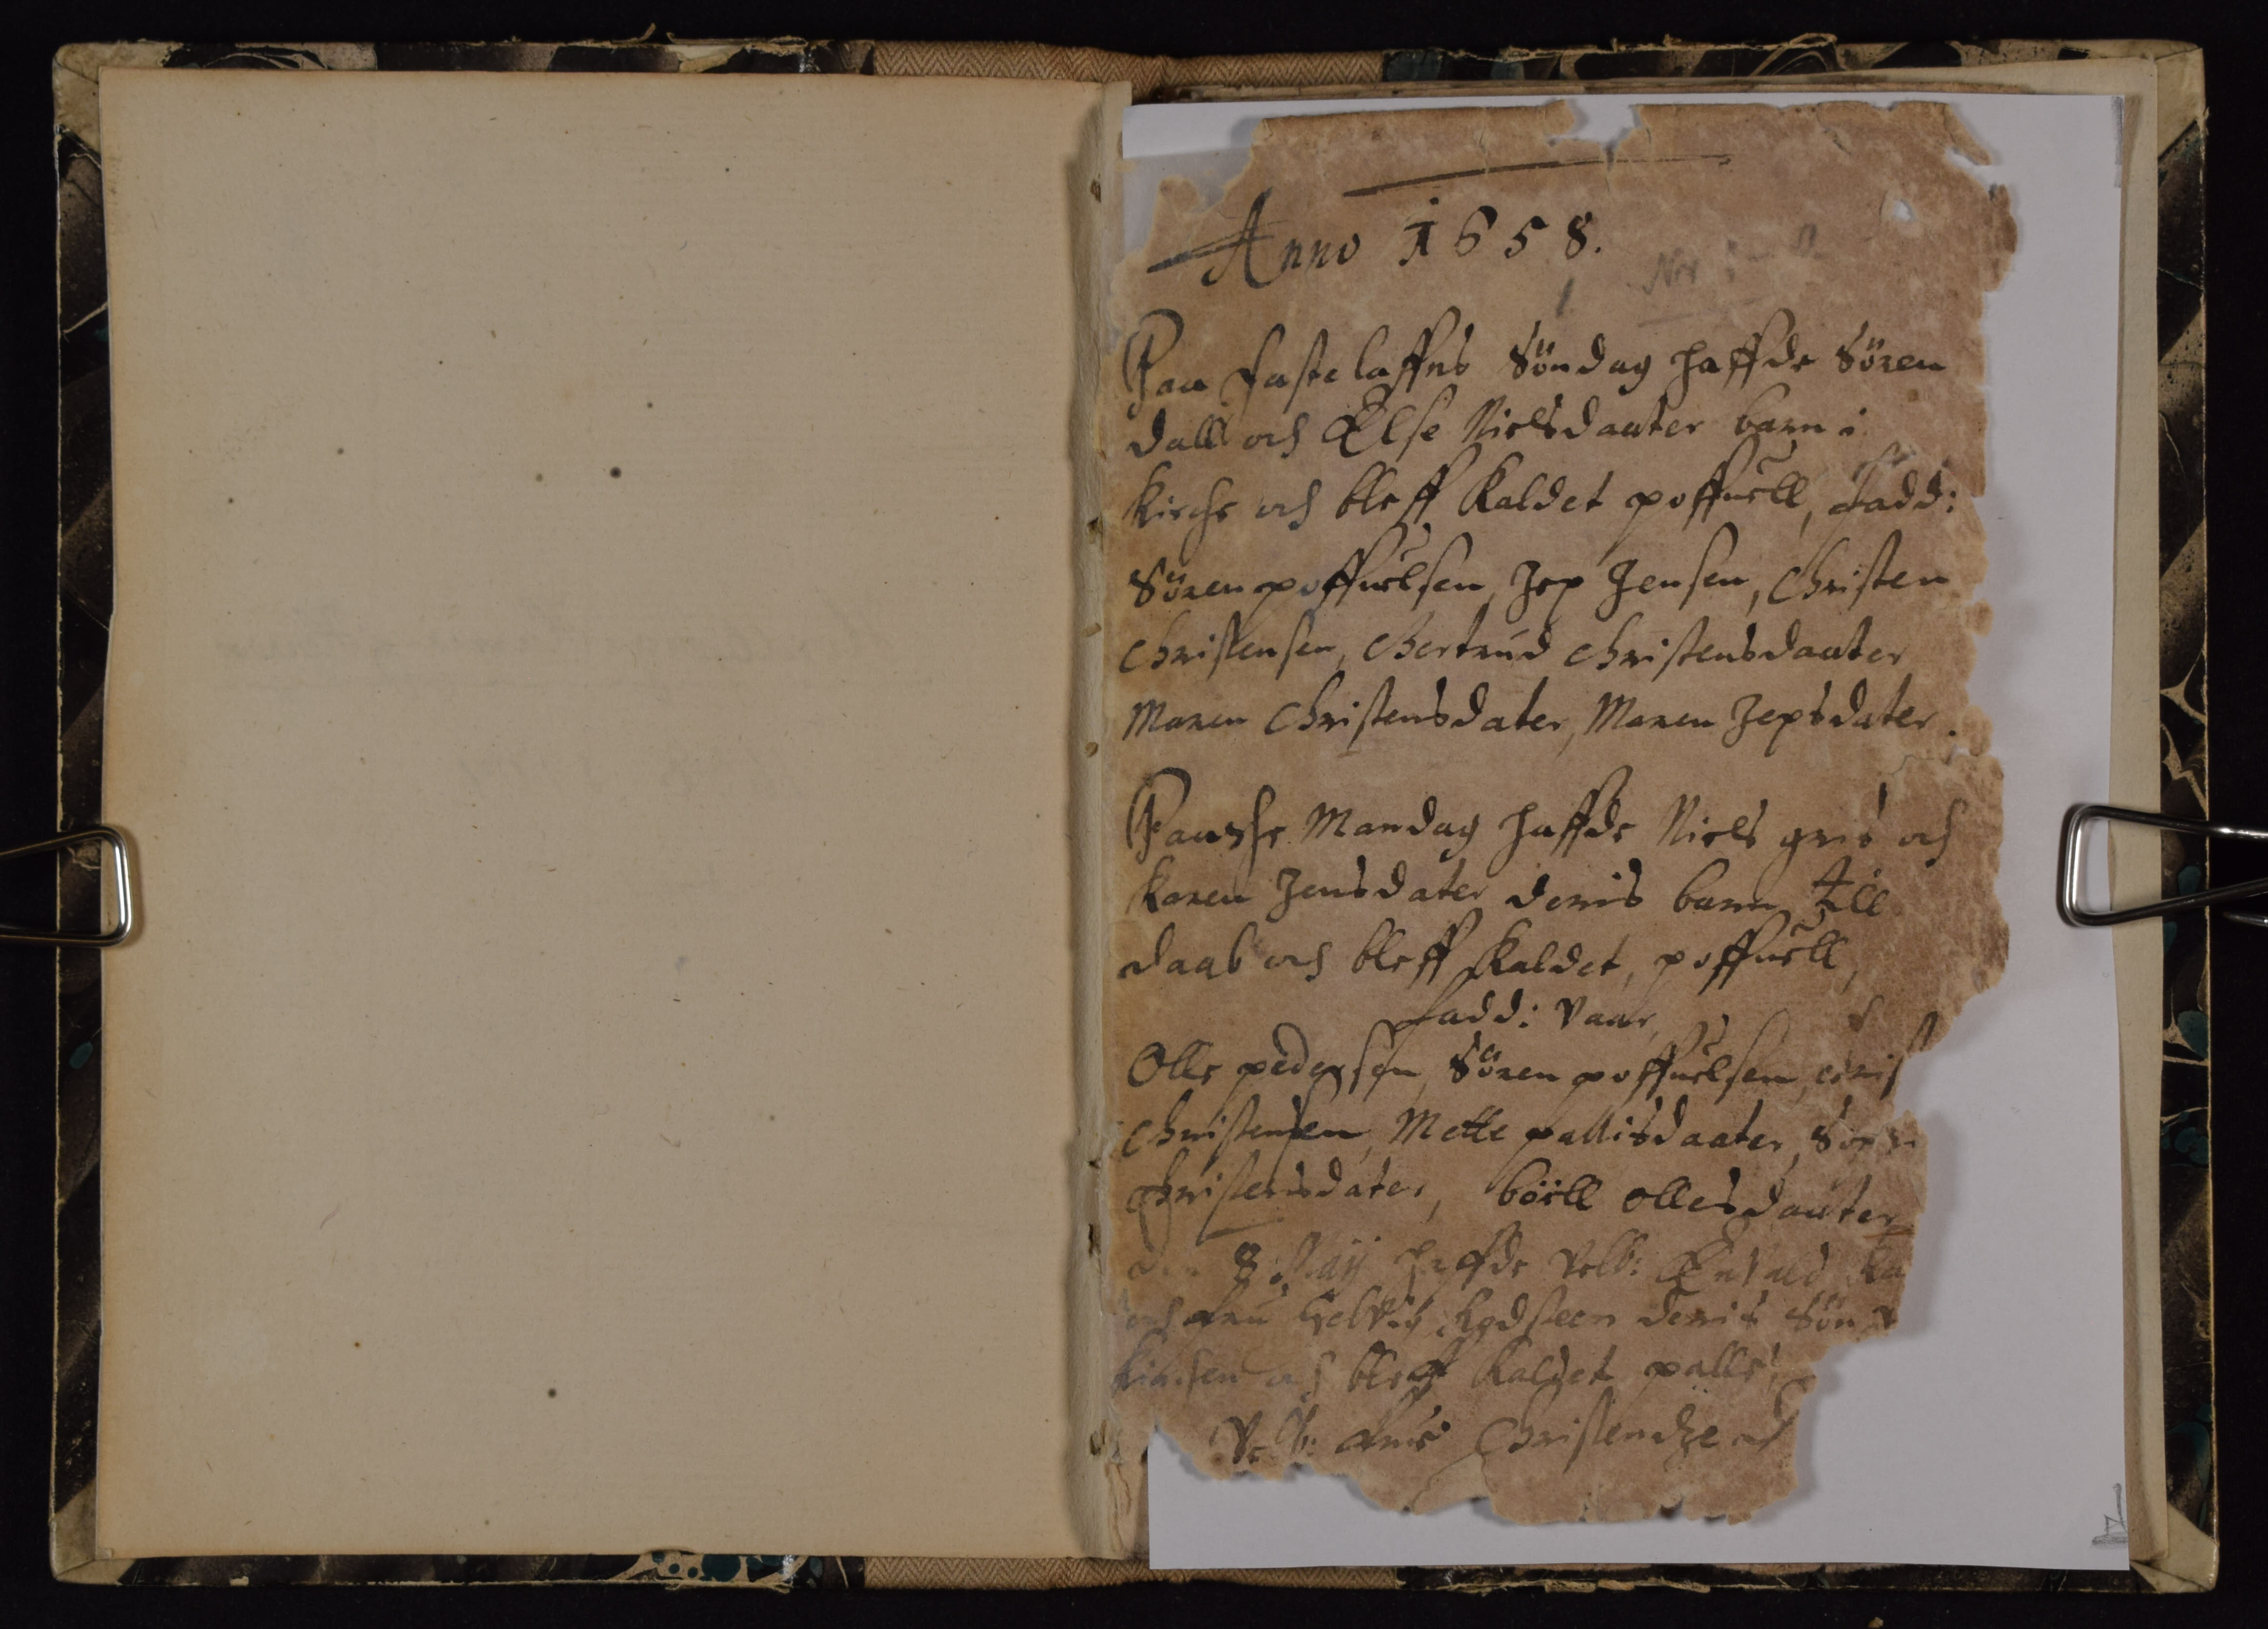Anno 1658.
Paa Fastelaffns Søndag haffde Søren
Dall och Else Nielsdauter barn i
Kirche och bleff kaldet poffuell Fadd:
Søren Poffuelsen, Jep Jensen, Christen
Christensen, Gertrud Christensdaater
Maren Christensdater, Maren Jepsdater
Pasche Mandag haffde Niels gr## och
Karen Jensdater deris barn till
daab och bleff kaldet, poffuell
Fadd: vaar
Olle pedersen Søren poffuelsen ##
Christensen Mette pallisdaater Sop##
Christensdater, boell Ollesdauter
Den 8 Maij hafde Velb: Envold Ka
och fru Helvig Radsteen deris Søn
Kirchen och bleff kaldet palle
Velb: fru Christendze
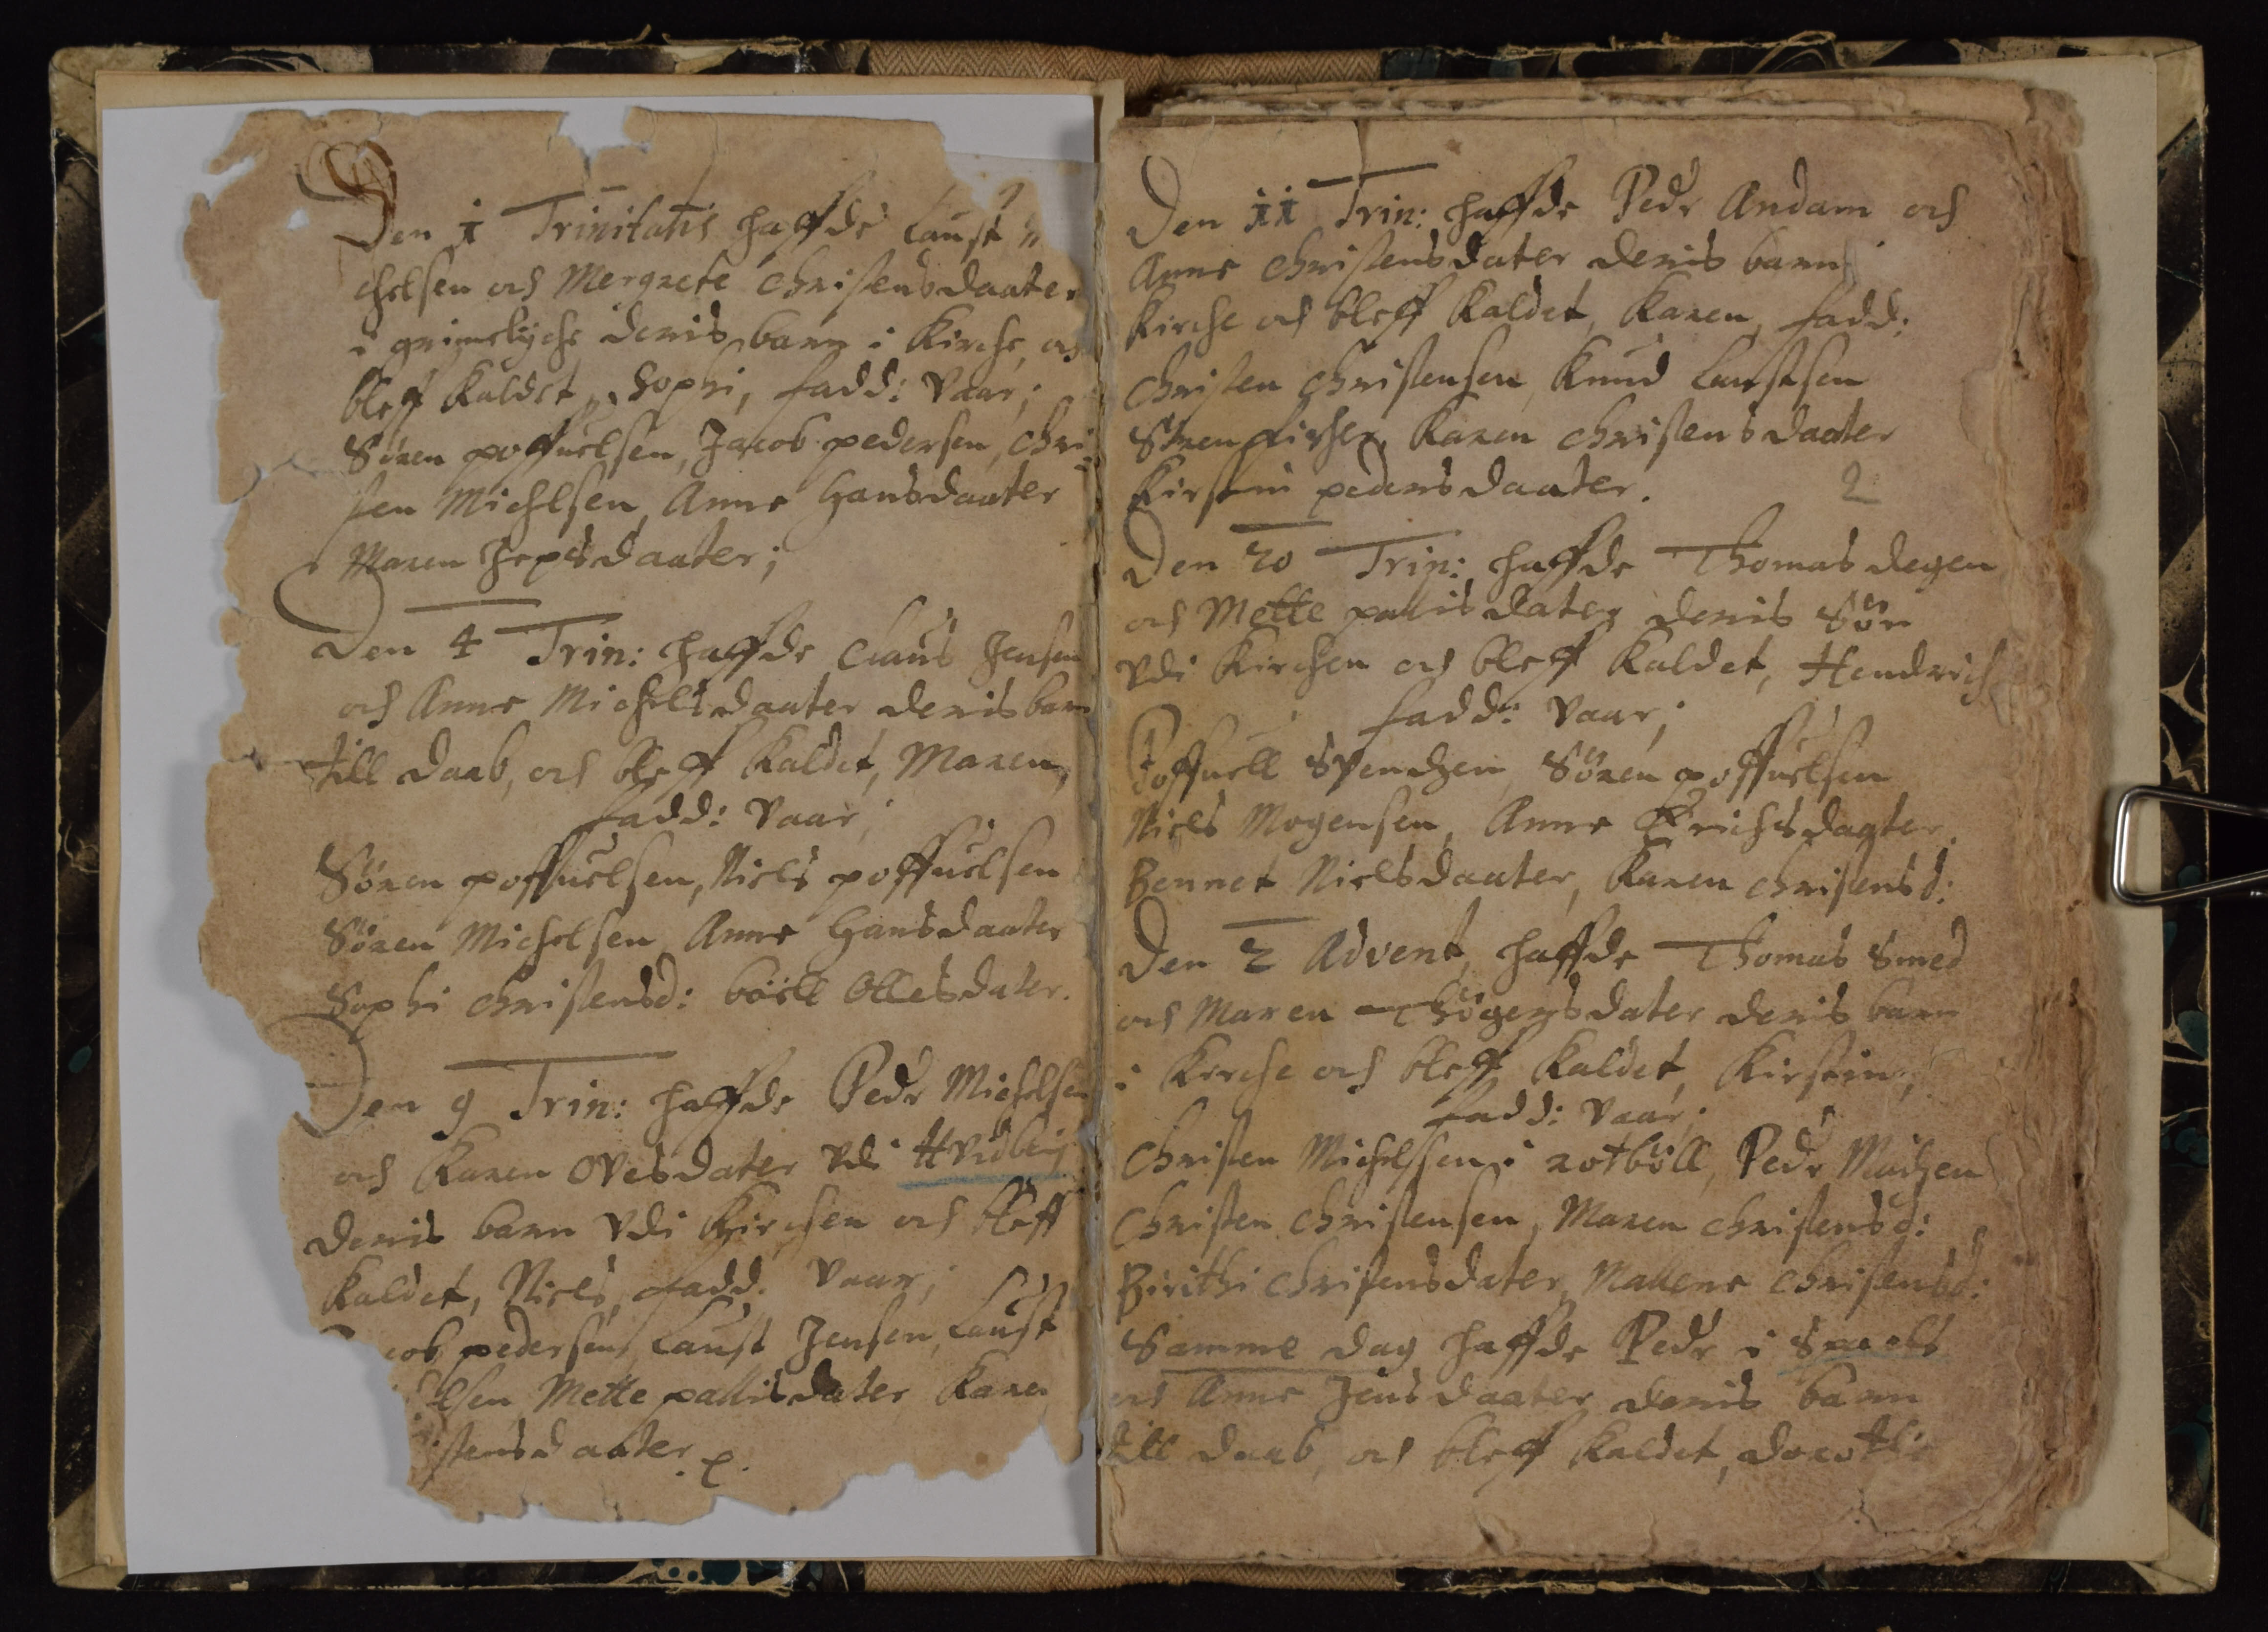Dom 1 Trinitatis haffde Laust
chelsen och Mergrete Christensdaater
i grimslyche deris barn i Kirche och
bleff kaldet Sophi, Fadd: vaar;
Søren Poffuelsen Jacob pedersen chri¬
sten Michelsen Anne Hansdaater
Maren Jepsdaater;
Den 4 Trin: haffde Claus Jensen
och Anne Michelsdauter deris barn
till Daab, och bleff kaldet, Maren
Fadd: vaar
Søren poffuelsen, Niels poffuelsen
Søren Michelsen Anne Hansdaater
Jophi Christensd: Boell Ollesdater
Don 9 Trin: haffde Pedr Michelsen
och Karen Ovesdatter vdi Hvidberg
deris barn vdi Kirchen och bleff
kaldet, Niels Fadd. vaar;
 cob pedersens Laust Jensen Laust
 elsen Mette Pallisdater Karen
 stensdaater
2
Don 11 Trin: haffde Pedr Andam och
Anne Christensdater deris barn
Kirche och bleff kaldet, Karen, Fadd:
Christen Christensen, Knud Laustsen
Søren Ficher Karen Christensdaatter
Kirsten Pedersdaater
Den 20 Trin: haffde Thomas Degen
och Mette pallisdater deris Søn
vdi Kirchen oc bleff kaldet, Hendrich
Fadd: vaar;
Poffuel Svendzen Søren poffuelsøn
Niels Mogensen, Anne Erichsdaater
Bennet Nielsdauter, Karen Christensd:
Den 2 Advent, haffde Thomas Smed
och Maren Thøgersdater deris barn
i Kirche och bleff kaldet Kirstin
Fadd: vaar;
Christen Michelsen i rotbøll, Pedr Madzen
Christen Christensen, Maren Christensd:
Birithe Christensdater Malene Christensd:
Samme dag haffde Pedr i Spaels
och Anne Jensdaater deris barne
till Daab och bleff kaldet Dorethe